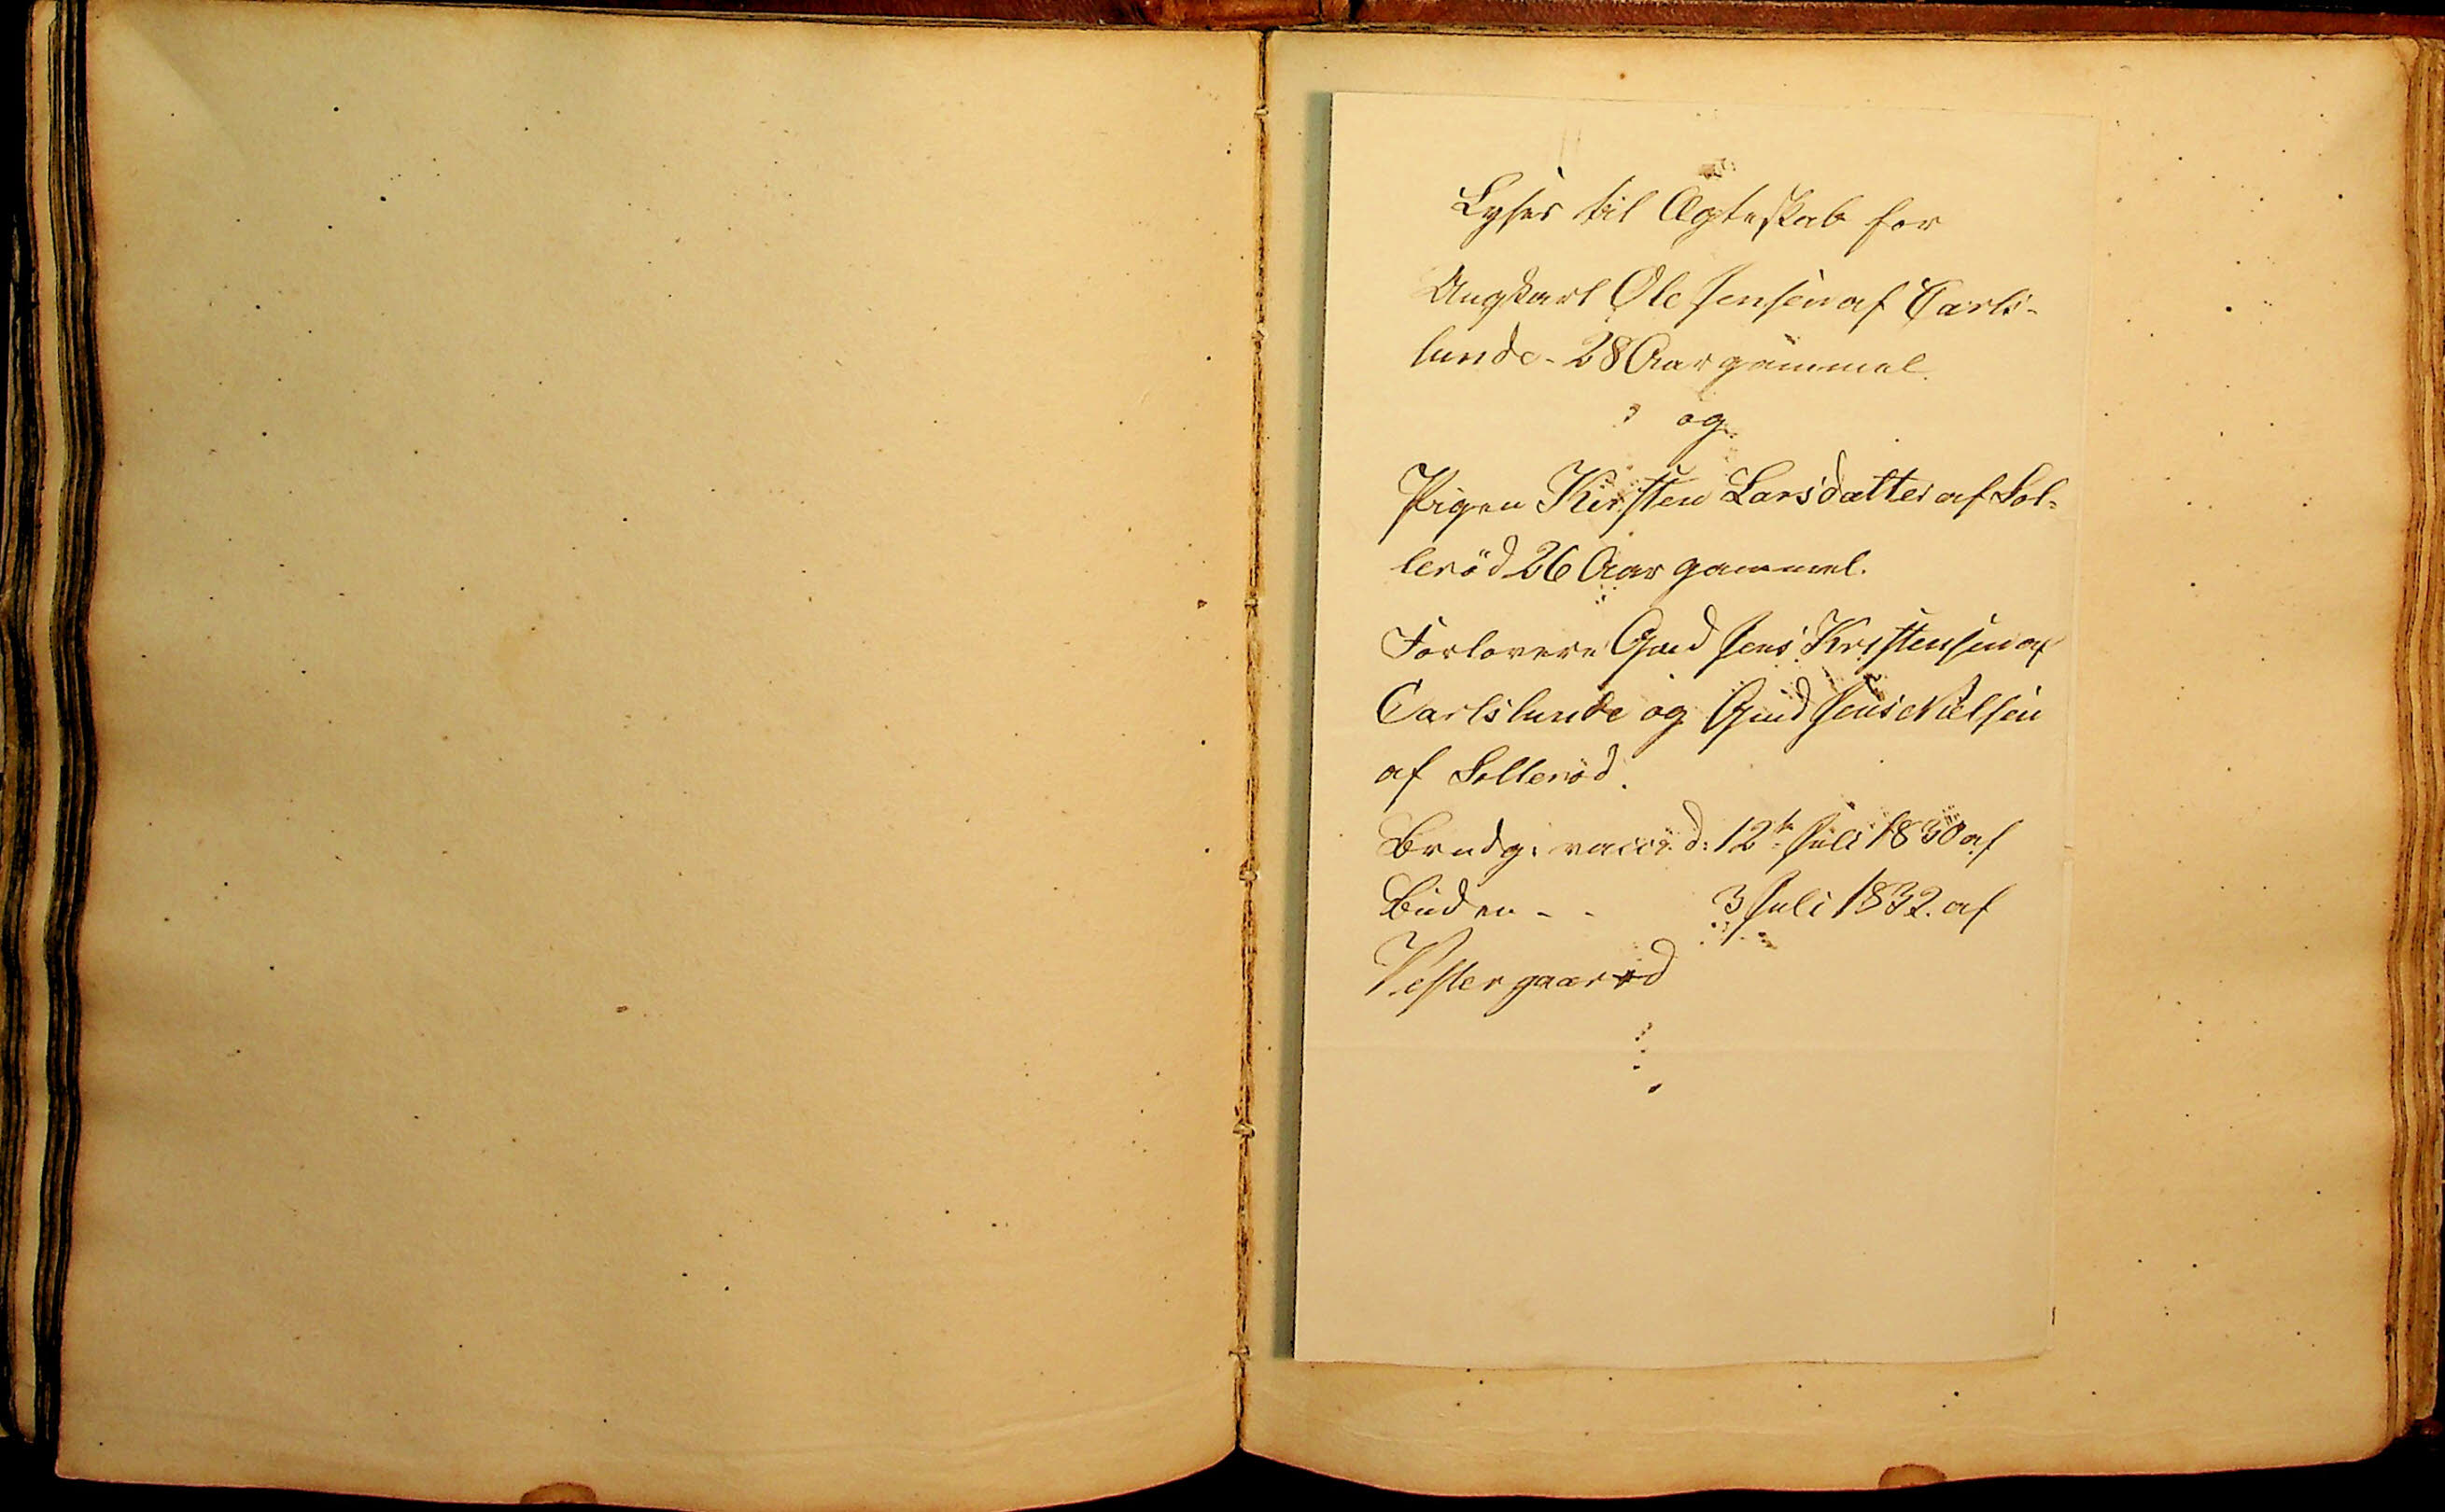Lyses til Ægteskab for
Ungkarl Ole Jensen af Carls-
lunde.   28 Aar gammel.
og
Pigen Kirstine Larsdatter af Sol¬
lerød 26 Aar gammel.
Forlovere Gmd Jens Kristensen af
Carlslunde og Gmd Jens Nielsen
af Sollerød.
Brudg: vacci. d: 12te Juli 1830 af
Bruden      
3 Juli 1832. af 
Vestergaarrd